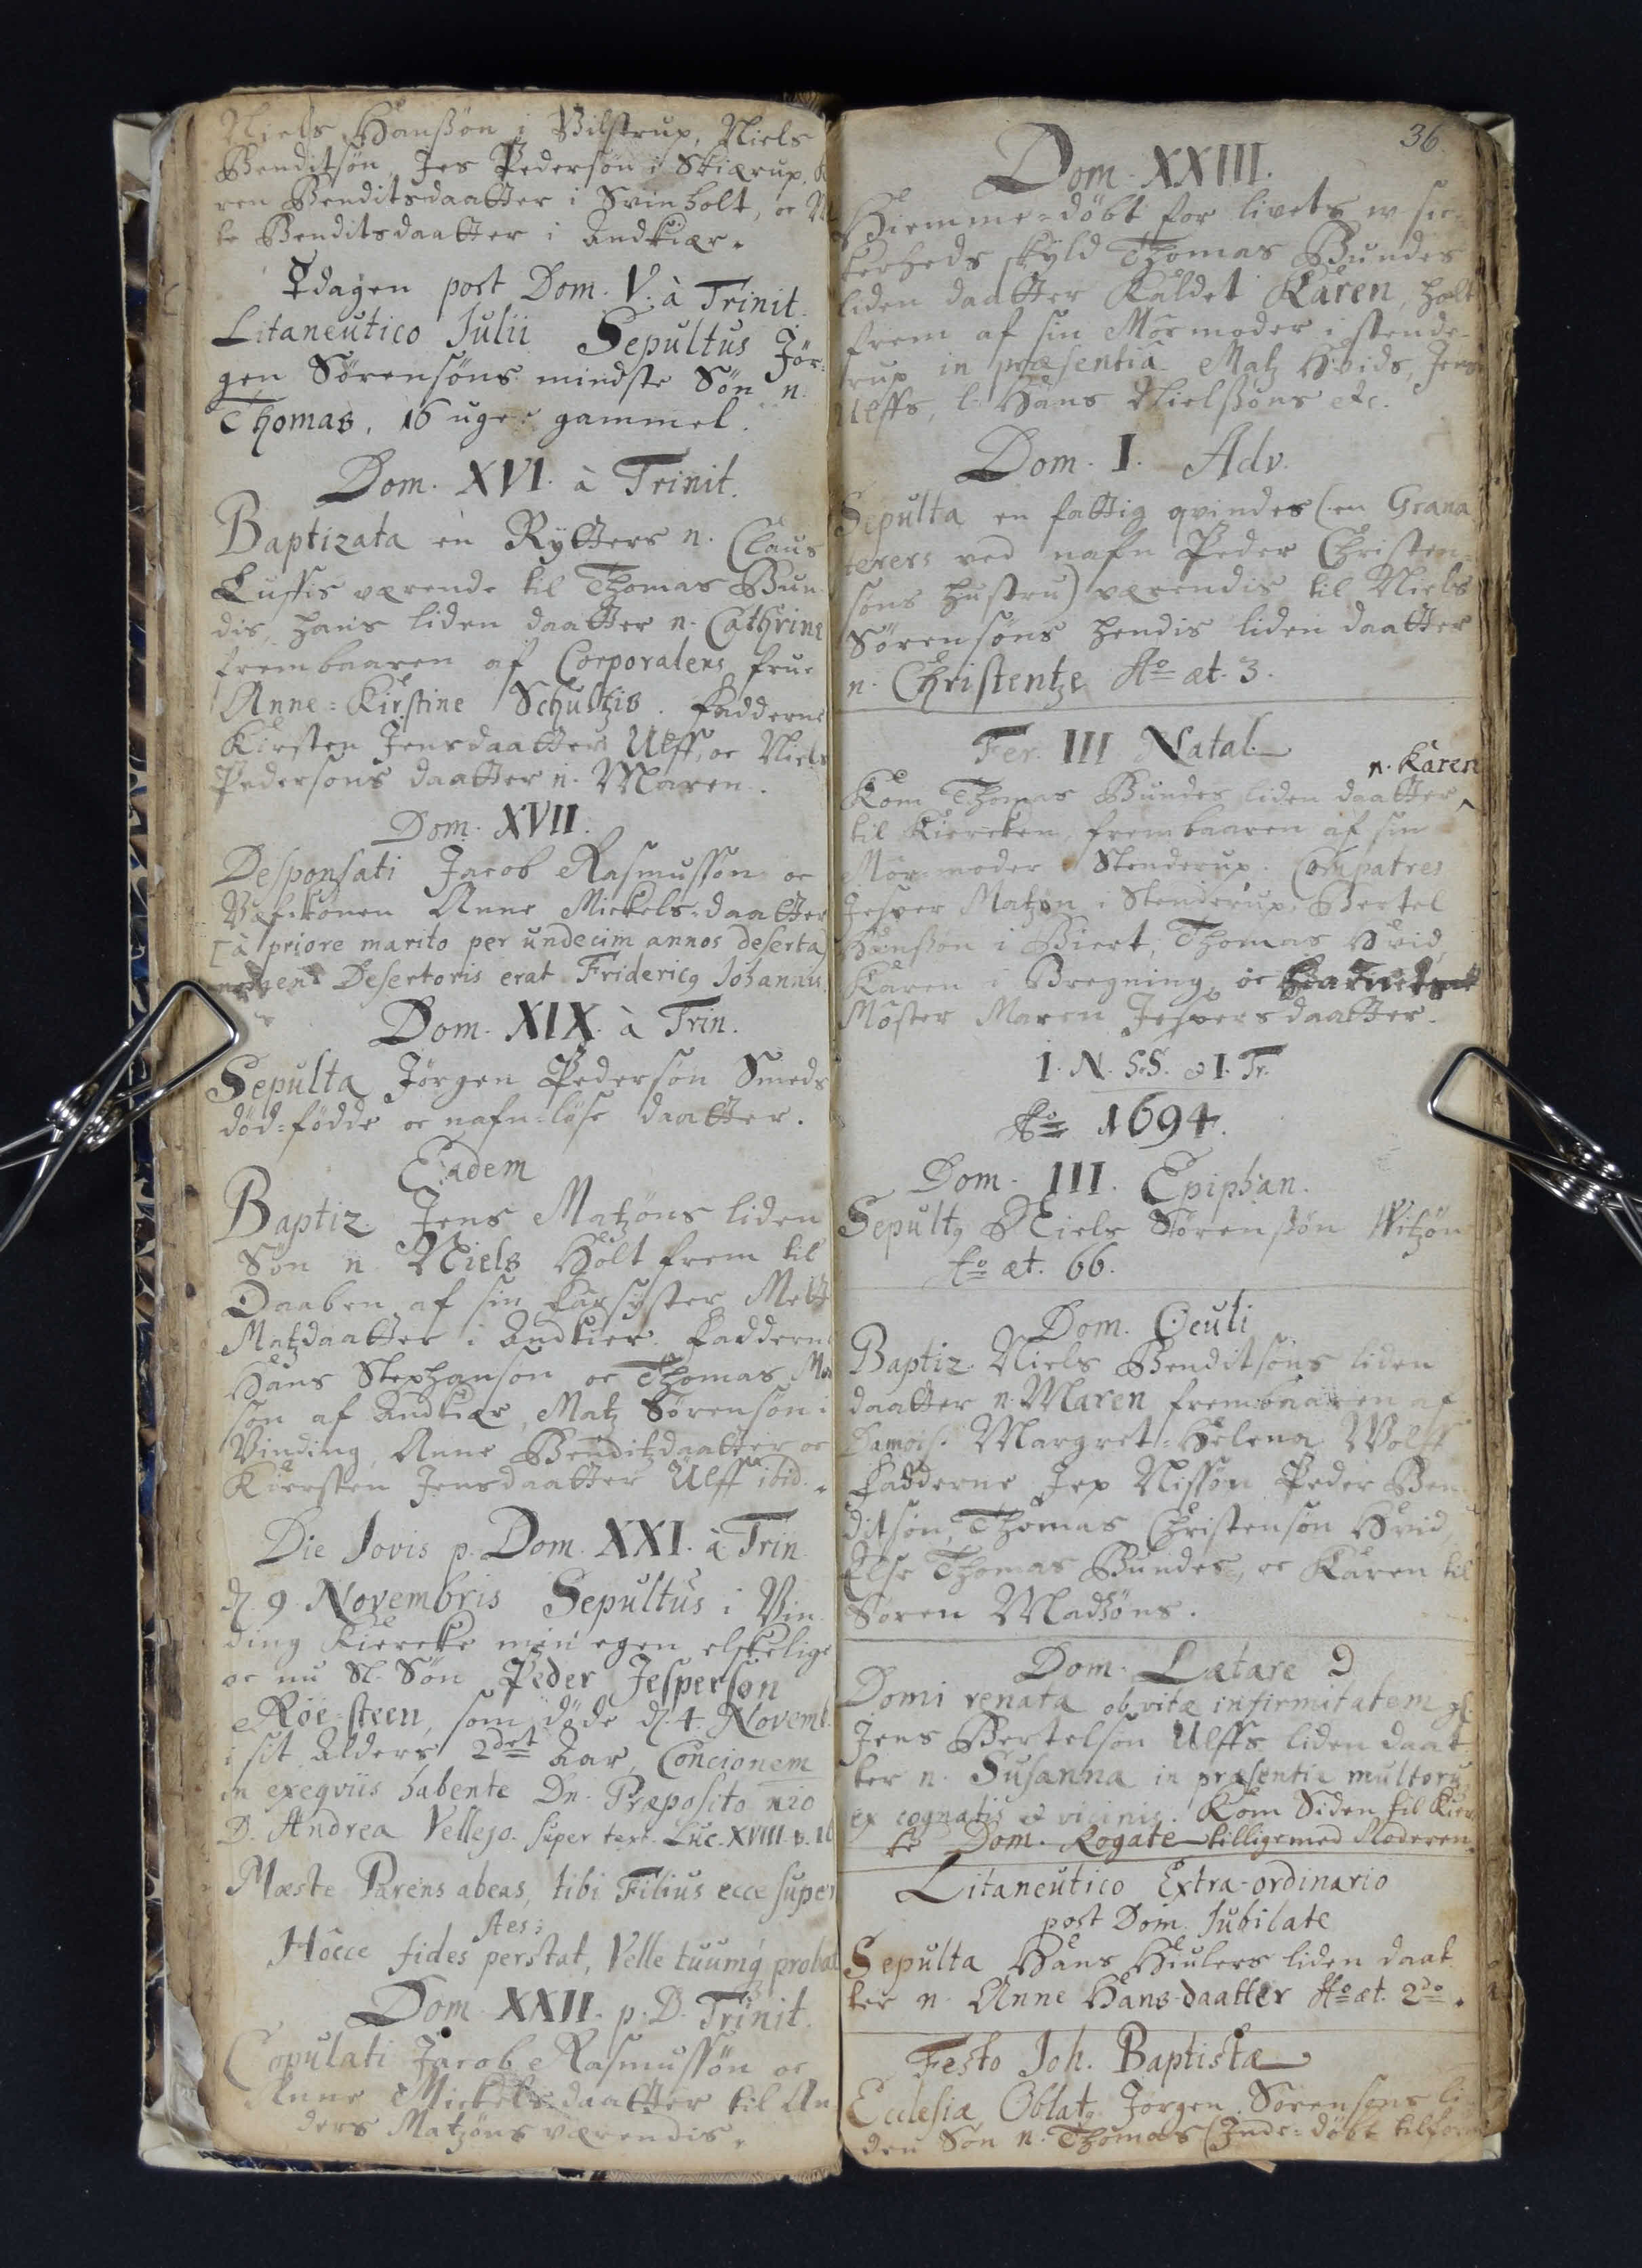Niels Hanssøn i Vilstrup, Niels
Benditsøn, Jes Pedersøn i Skiærup,
ren Benditsdaatter i Svinholt, oc
te Benditsdaatter i Andkiær.
☿ dagen post Dom. V a Trinit.
Litaneutico Julii. Sepultus Jør¬
gen Sørensøns mindste Søn n.
Thomas, 16 uger gammel
Dom. XVI a Trinit.
Baptizata en Rytters n. Claus
Luffis værende til Thomas Bun¬
des, hans liden daatter n. Cathrine
frembaaren af Corporalens frue
Anne=Kirstine Schultzis. Faddere
Kirsten Jensdatter Ulff, oc Niels
Pedersøns daatter n. Maren
Dom. XVII.
Desponsati Jacob Rasmussøn oc
Væfekonen Anne Mickels = daatter
[a priore marito per undecim annos deserta)
Desertoris erat Fridericq Johannis.
Dom. XIX a Trin. 
Sepulta Jørgen Pedersøn Smeds
død=fødde oc nafn=løse daatter.
Eodem
Baptiz Jens Matzøns liden
Søn n. Niels, holt frem til
Daaben af sin Farsyster Mette
Matzdaatter i Andkier. Fadderne
Hans Stephansøn oc Thomas Ma
søn af Andkiær, Matz Sørensøn i
Vinding, Anne Benditzdaatter oc
Kiersten Jensdaatter Ulff ibid.
Die Jovis p. Dom. XXI a Trin
d. 9 Novembris. Sepultus i Vin¬
ding Kiercke min egen elskelige
oc nu Sl. Søn Peder Jespersøn 
Roe=steen, som døde d. 4 Novemb.
sit Alders 2det Aar, Concionem
in exeqviis habente Dn. Præpasito nio
D. Andrea Vellejo. super text Lkc. XVIII p.16.
Mæste Parens abeas, tibi Filius ecce super
Aess##
Hocce fides perstat, Velle tuumq proba
Dom. XXII p. D. Trinit
Copulati Jacob Rasmussøn oc
Anne Mickelsdaatter til An
ders Matzøns værendis.
36
Dom. XXIII.
Hiemme=døbt for livets v-si¬
kerheds skyld Thomas Bundes
liden daatter kaldet Karen, holt
frem af sin Mor moder i stende¬
rup, in præsentia Matz Hvids, Jens
Ulffs, l. Hans Nielssøns etc
Dom. I. Adv.
Sepulta en fattig qvindes (en Grana
terers ved nafn Peder Christen¬
søns hustru) værendis til Niels
Sørensøns hendis liden daatter 
n. Christentze Ao æt. 3
Fer. III Natal.
n. Karen
Kom Thomas Bundes liden daatter
til Kiercken, frembaaren af sin
Mor=moder i Stenderup. Compatres
Jesper Matzøn i Stenderup. Bertel
Hanssøn i Biert. Thomas Hvid.
Moster Maren Jespersdaatter.
Karen i Bregning oc
I.N.S.S ## I. Tr.
Ao 1694
Dom. III Epiphan
Sepultq Niels Sørensøn Witzøn
Ao æt. 66
Dom. Oculi
Baptiz Niels Benditsøns liden
daatter n. Maren, frembaaren af 
Margret=Helena Wolff
Fadderne Jep Nissøn. Peder Ben
ditsøn, Thomas Christensøn Hvid
Else Thomas Bundes, oc Karen til
Søren Madtzøns
Dom. Lætare d
Domi renata ob vitæ infirmitatem gl. 
Jens Bertelsøn Ulffs liden daat
ter n. Susanna, in præsentia
ex cognatis et vicinis. Kom siden til
ke Dom. Rogate tilligemed Moderen
Litaneutico Extra- ordinario
post Dom. Jubilate
Sepulta Hans Hiulers liden daat¬
ter n. Anne Hansdaatter Ao æt. 2do.
Festo Joh. Baptistæ
Ecclesiæ Oblatq. Jørgen Sørensøns li
den Søn n. Thomas (Inde=døbt
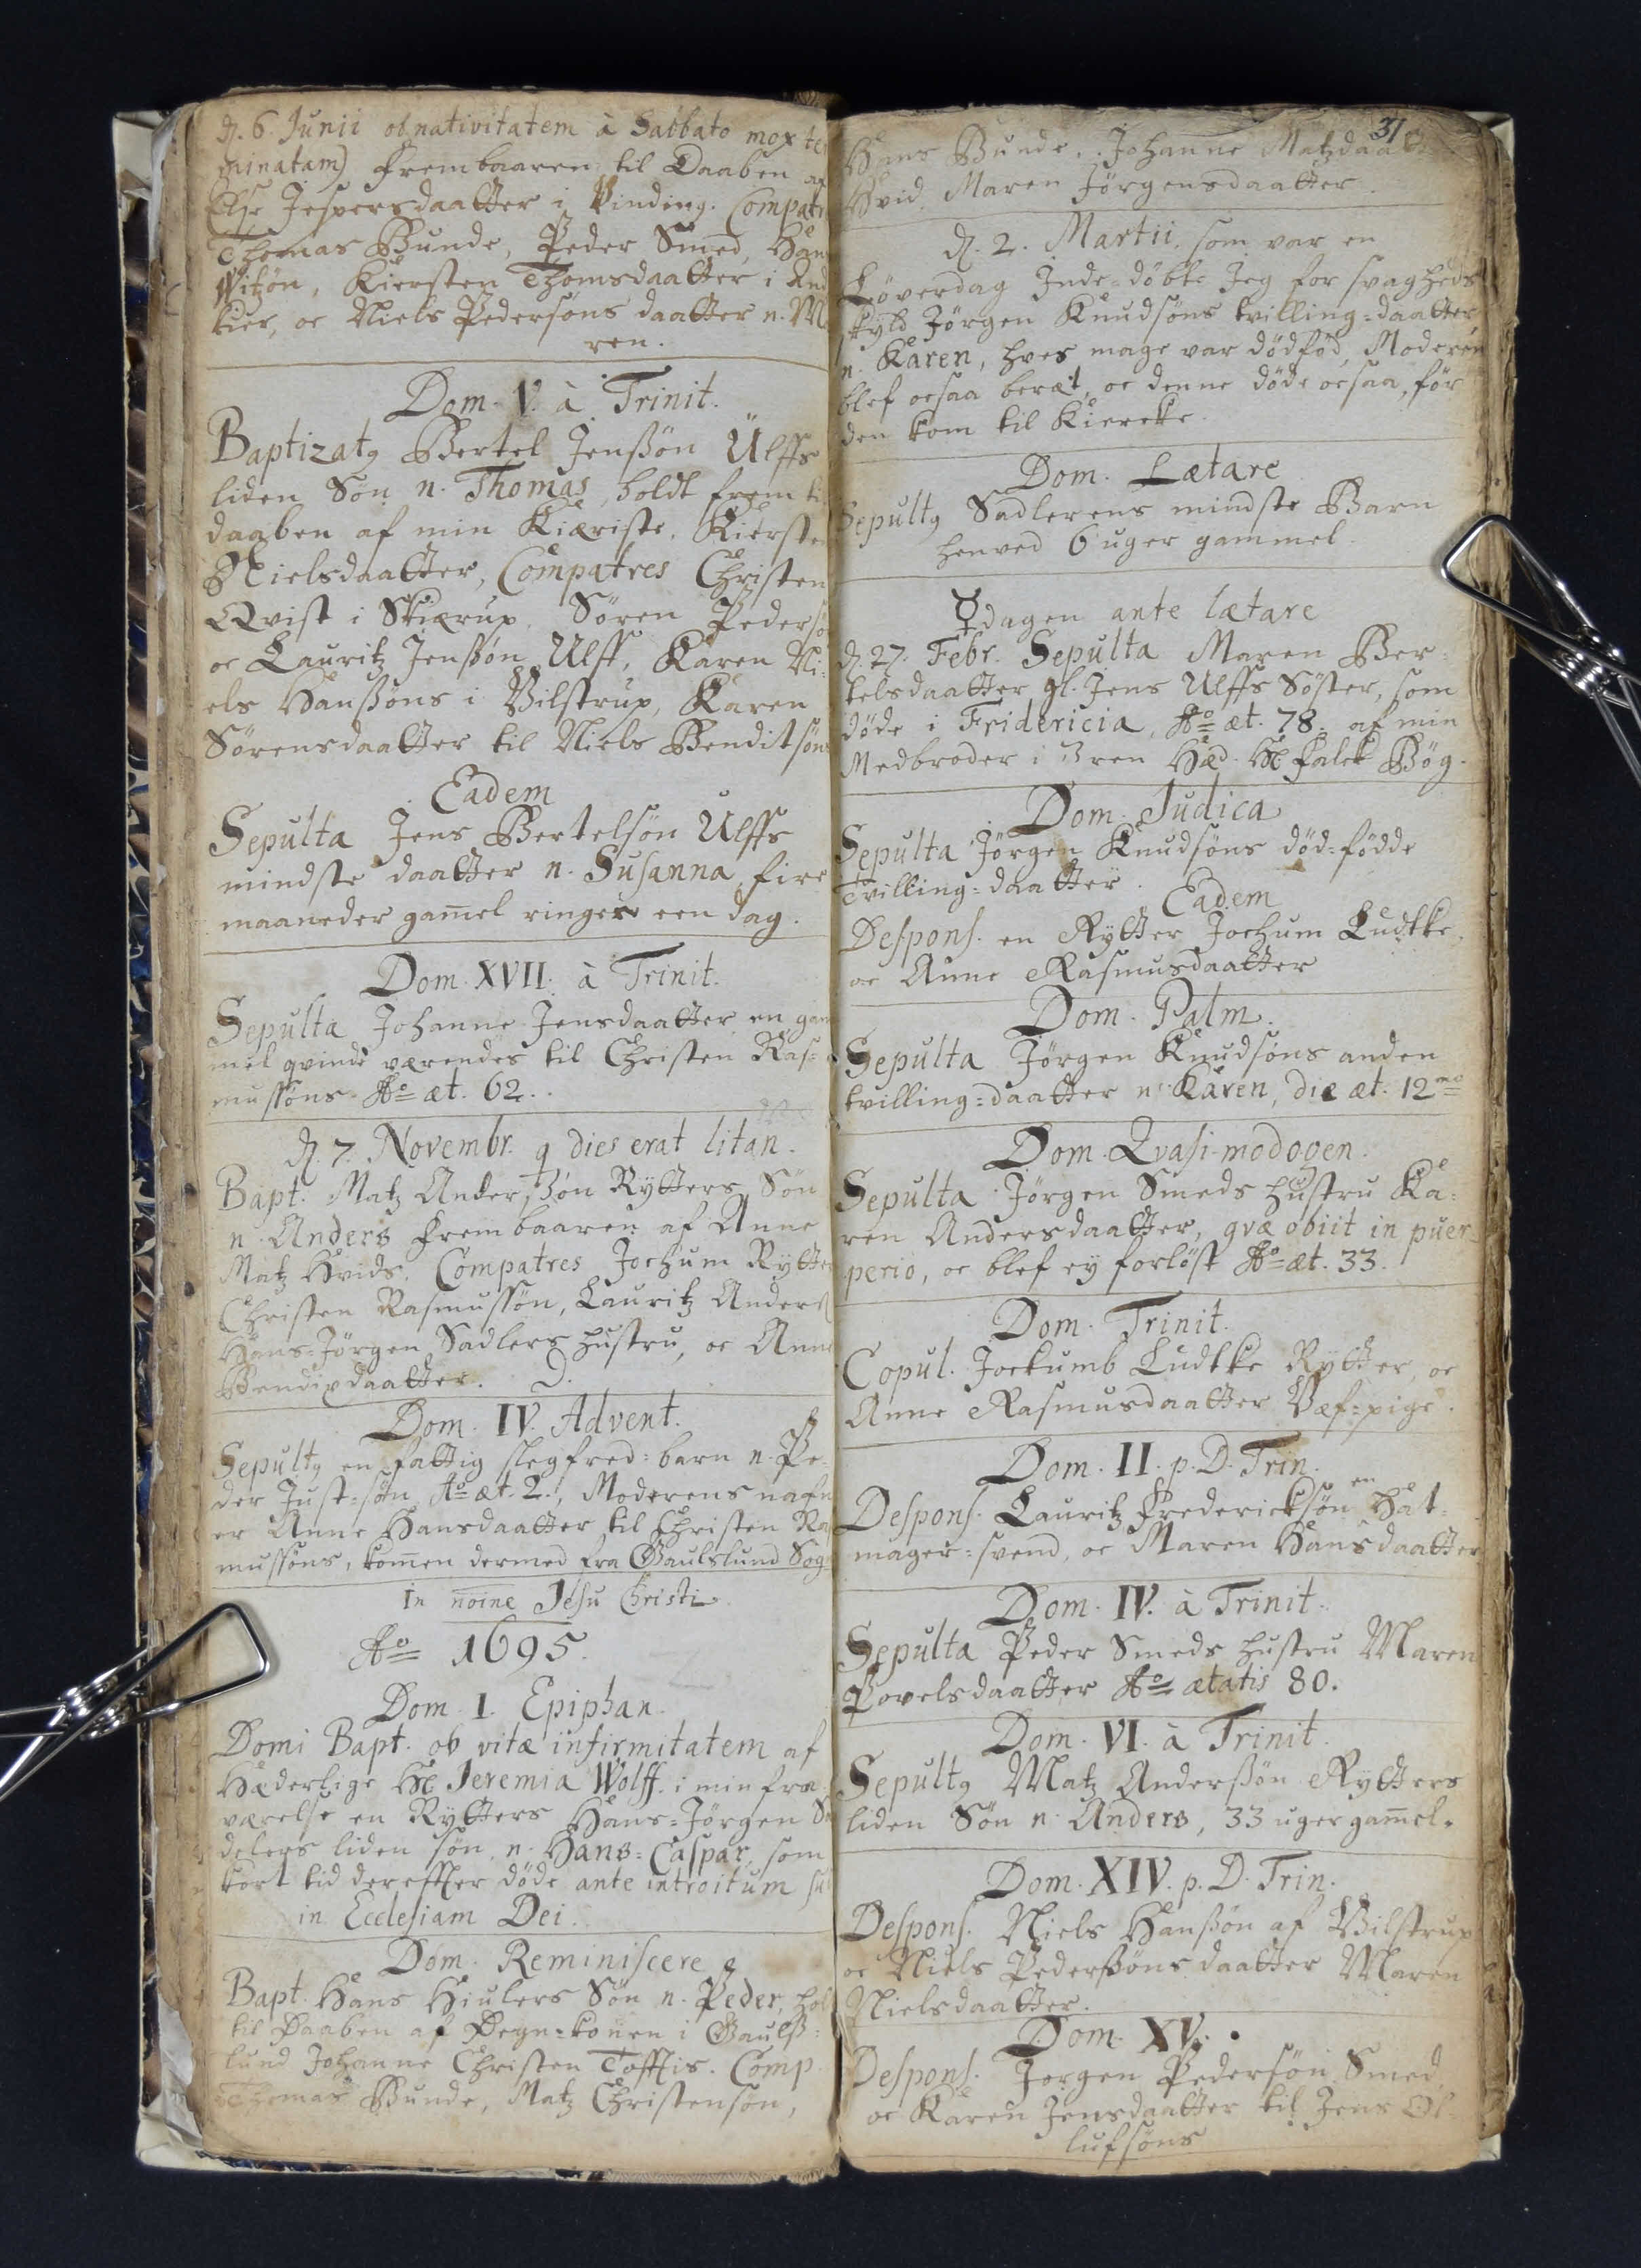d. 6 Junii obnativitatem a Sabbato mox ter
minatam) frembaaren til Daaben af 
Else Jespersdaatter i Vinding. Compatres
Thomas Bunde, Peder Smed. Hans
Witzøn, Kiersten Thomasdaatter i And
kier, oc Niels Pedersøns daatter n. M 
ren.
Dom. V a Trinit.
Baptizatq Bertel Jenssøn Ulffs
liden Søn n. Thomas, holdt frem ti 
daaben af min kiæriste Kiersten 
Nielsdaatter, Compatres Christen
Qvist i Skiærup. Søren Pedersø 
oc Lauritz Jenssøn Ulff,. Karen Ni¬
els Hanssøns i Vilstrup, Karen
Sørensdaatter til Niels Benditsøns
Eodem
Sepulta Jens Bertelsøn Ulffs 
mindste daatter n. Susanna fire 
maaneder gammel ringere een dag
Dom. XVII a Trinit.
Sepulta Johanne Jensdaatter en gam
mel qvinde værendes til Christen Ras¬
mussøns Ao æt. 62
d. 7 Novembr. q dies erat litan.
Bapt. Matz Ander, søn Rytters Søn
n. Anders frembaaren af Anne
Matz Hvids. Compatres. Jochum Rytter
Christen Rasmussøn, Lauritz Anders
Hans=Jørgen Sadlers hustru, oc Anne
Benditsdaatter.
Dom. IV. Advent
Sepultg en fattig slegfred=barn n. Pe¬
der Just=søn Ao æt. 2., Moderens nafn
er Anne Hansdaatter til Christen Ras
mussøns, komen dermed fra Gaulslund Sogn
In noine Jesu Christi
Ao 1695
Dom. 1. Epiphan
Domi Bapt. ob vitæ infirmitatem af
Hæderlige Hl. Jeremia Wolff i min fra
værelse en Rytters Hans=Jørgen Sa
delers liden søn n. Hans=Caspar, som
kort tid dereffter døde ante introitum sui
in Ecclesiam Dei
Dom. Reminiscere
Bapt. Hans Hiulers Søn n. Peder, hol 
til Daaben af Degne=Konen i Gaulss¬
lund Johanne Christen Tofftis. Comp.
Thomas Bunde, Matz Christensøn
31
Hans Bunde. Johanne Matzdaa
Hvid. Maren Jørgensdaatter
d. 2. Martii, som var en
Løverdag Inde=døbte Jeg for svagheds
skyld Jørgen Knudsøns tvilling=daatter
n. Karen, hves mage var dødfød, Moderen
blef ocsaa beræt, oc denne døde ocsaa før 
den kom til Kiercke
Dom. Lætare
Sepultq Sadlerens mindste Barn
henved 6 uger gammel.
☿ dagen ante Lætare
d. 27 Febr. Sepulta Maren Ber¬
telsdaatter gl. Jens Ulffs Søster, som
døde i Fridericia, Ao æt. 78. af min
Medbroder i ##ren Hæd. Hl. Falck Bøg
Dom: Judica
Sepulta Jørgen Knudsøns død=fødde
Tvilling=daatter.
Eodem
Despons. en Rytter Jochum Ludtke
oc Anne Rasmusdaatter
Dom. Palm.
Sepulta Jørgen Knudsøns anden
tvilling=daatter n. Karen, diæ æt. 12mo
Dom. Qvasi-modogen
Sepulta Jørgen Smeds hustru Ka¬
ren Andersdaatter, qvæ obiit in puer
perio, oc blef ey forløst Ao æt. 33.
Dom. Trinit.
Copul. Jockumb Ludtke Rytter oc
Anne Rasmusdaatter Væf=pige
Dom. II. p. D. Trin
en
Despons. Lauritz Fredericksøn en Hat¬
mager=svend, oc Maren Hansdaatter
Dom. IV. a Trinit
Sepulta Peder Smeds hustru Maren 
Povelsdaatter Ao ætatis 80.
Dom. VI. a Trinit
Sepultq Matz Anderssøn Rytters
liden Søn n. Anders, 33 uger gammel.
Dom. XIV. p. D. Trin
Despons. Niels Hanssøn af Vilstrup
oc Niels Pederssøns daatter Maren
Nielsdaatter
Dom. XV.
Despons. Jørgen Pedersøn Smed
oc Karen Jensdaatter til Jens Ol¬
lufsøns